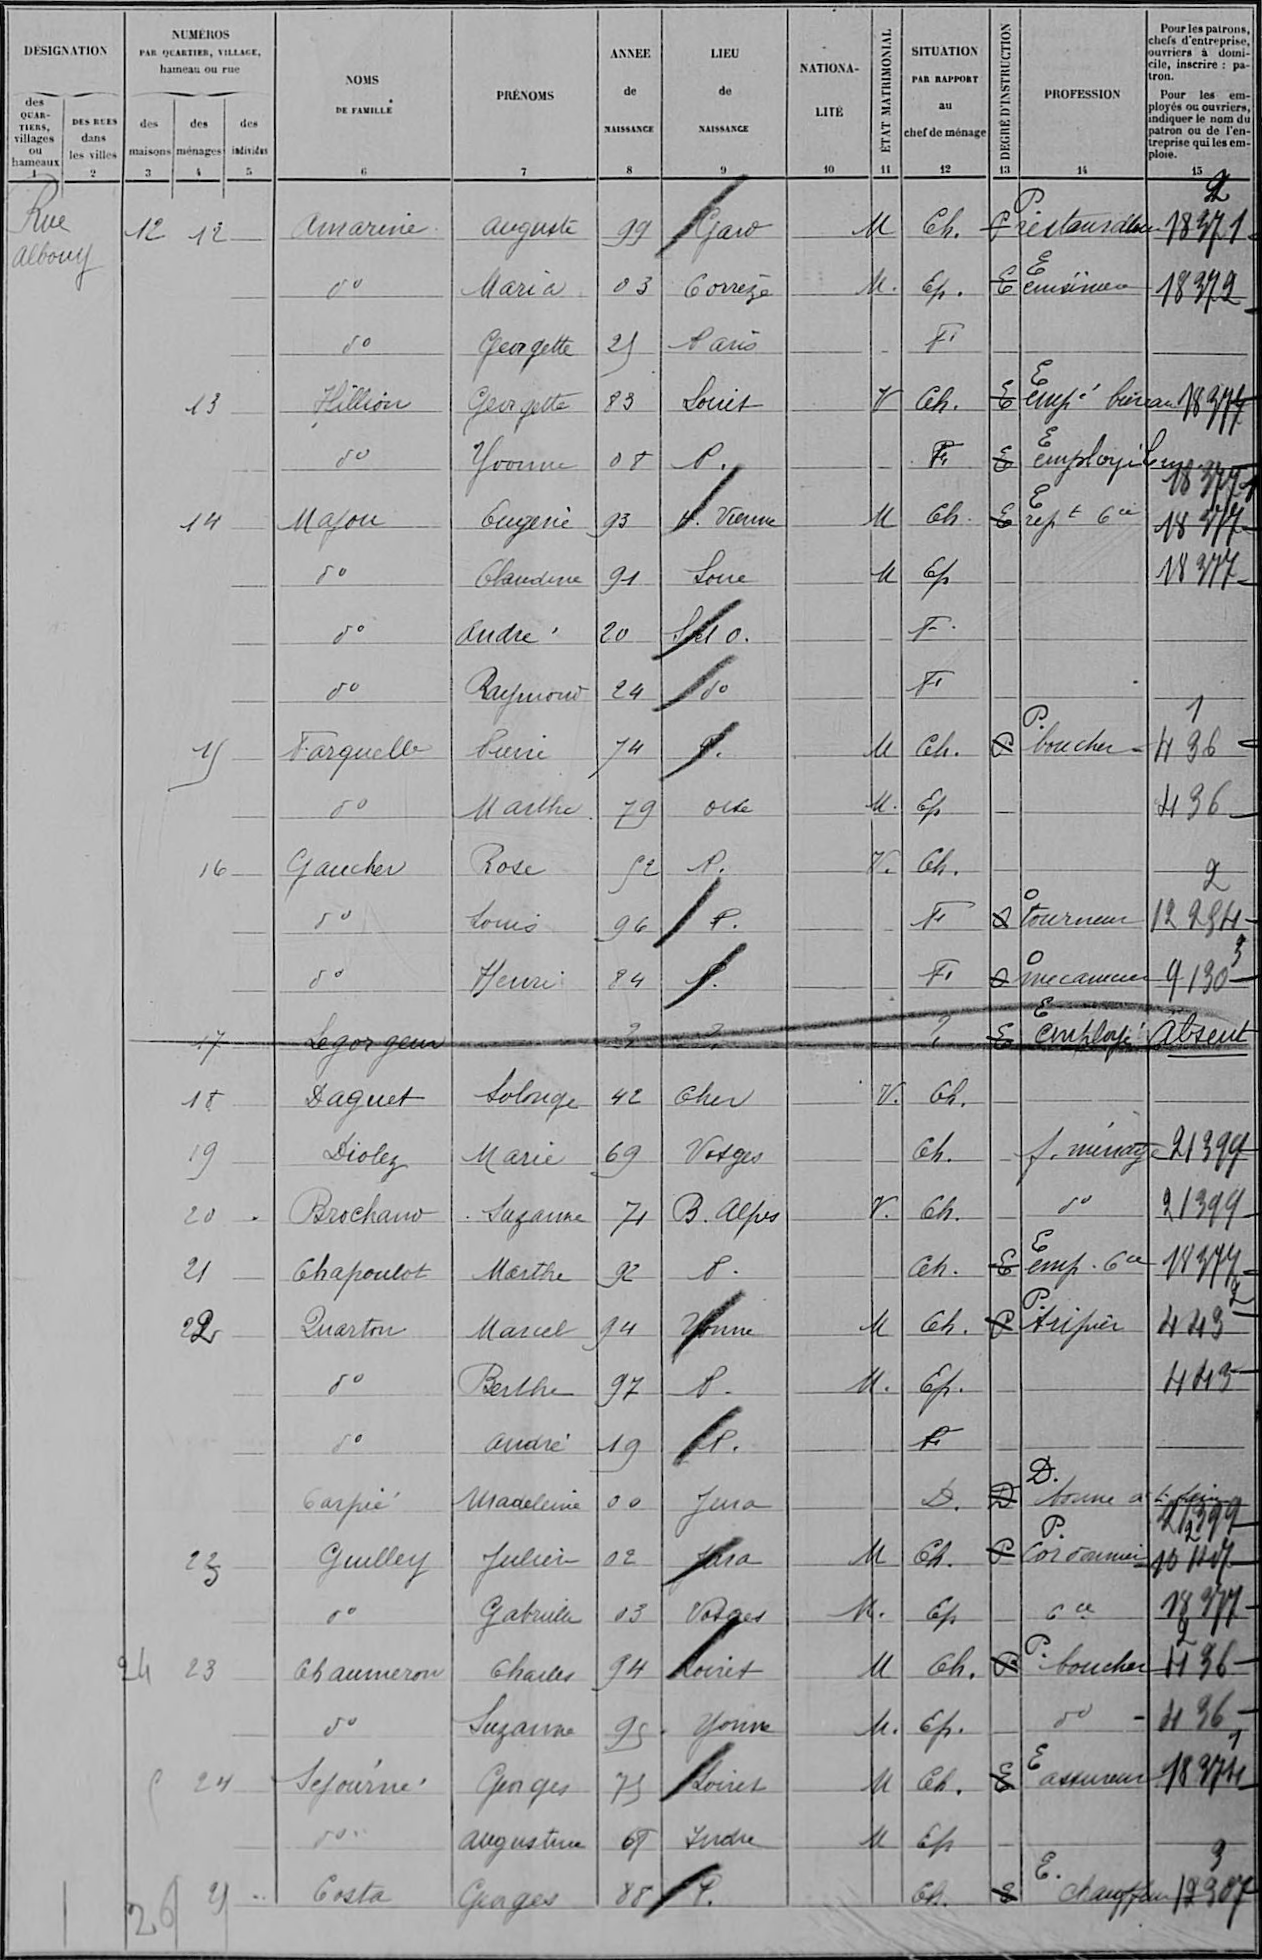Armarine/Auguste/99/Gard/¤/M/Ch /¤/P restaurateur/18371
d°/Maria/03/Corrèze/¤/M /Ep /¤/E cuisinière/18372
d°/Georgette/25/Paris/¤/¤/F/¤/¤/¤
Hillion/Georgette/83/Louis/¤/V/Ch /¤/E empé bureau/18377
d°/Yvonne/08/P /¤/¤/F/¤/E employée Cm/!18377
Majou/Eugène/93/H Vienne/¤/M/Ch /¤/E rept Cce/18377
d°/Claudine/91/Loire/¤/M/Ep/¤/¤/18377
d°/André/20/S et O /¤/¤/F/¤/¤/¤
d°/Raymond/24/d°/¤/¤/F/¤/¤/¤
Farquelle/Pierre/74/P /¤/M/Ch /¤/P boucher/436
d°/Marthe/79/Oise/¤/M /Ep/¤/¤/436
Gaucher/Rose/52/P /¤/V /Ch /¤/¤/¤
d°/Louis/96/P /¤/¤/F/¤/o tourneur/12254
d°/Henri/84/P /¤/¤/F /¤/o mecanicien/9130
Legorgeur/¤/¤/¤/¤/¤/¤/¤/E Employé/absent
Daguet/Solange/42/Cher/¤/V /Ch /¤/¤/¤
Diolez/Marie/69/Vosges/¤/¤/Ch /¤/f ménage/21399
Brochand/Suzanne/71/B Alpes/¤/V /Ch /¤/d°/21399
Chapoulot/Marthe/92/P /¤/¤/Ch /¤/E emp Cce/18377
Quarton/MArcel/94/Yonne/¤/M/Ch /¤/P tripier/443
d°/Berthe/97/P /¤/M /Ep /¤/¤/443
d°/André/10/P /¤/¤/f/¤/¤/¤
Tarpié/Madeleine/00/Jura/¤/¤/D /¤/D bonne à t faire/¤
Guilley/Julien/02/Jura/¤/M/Ch /¤/P cordonnier/10147
d°/Gabrielle/03/Vosges/¤/M /Ep/¤/cce/18377
Chaumeron/Charles/94/Loiret/¤/M/Ch /¤/P boucher/436
d°/Suzanne/95/Yonne/¤/M /Ep /¤/d°/436
Séjourné/Georges/75/Loiret/¤/M/Ch /¤/E assureur/18374
d°/augustine/68/Indre/¤/M/Ep/¤/¤/¤
Costa/Georges/88/P /¤/¤/Ch /¤/E chauffeur/12307
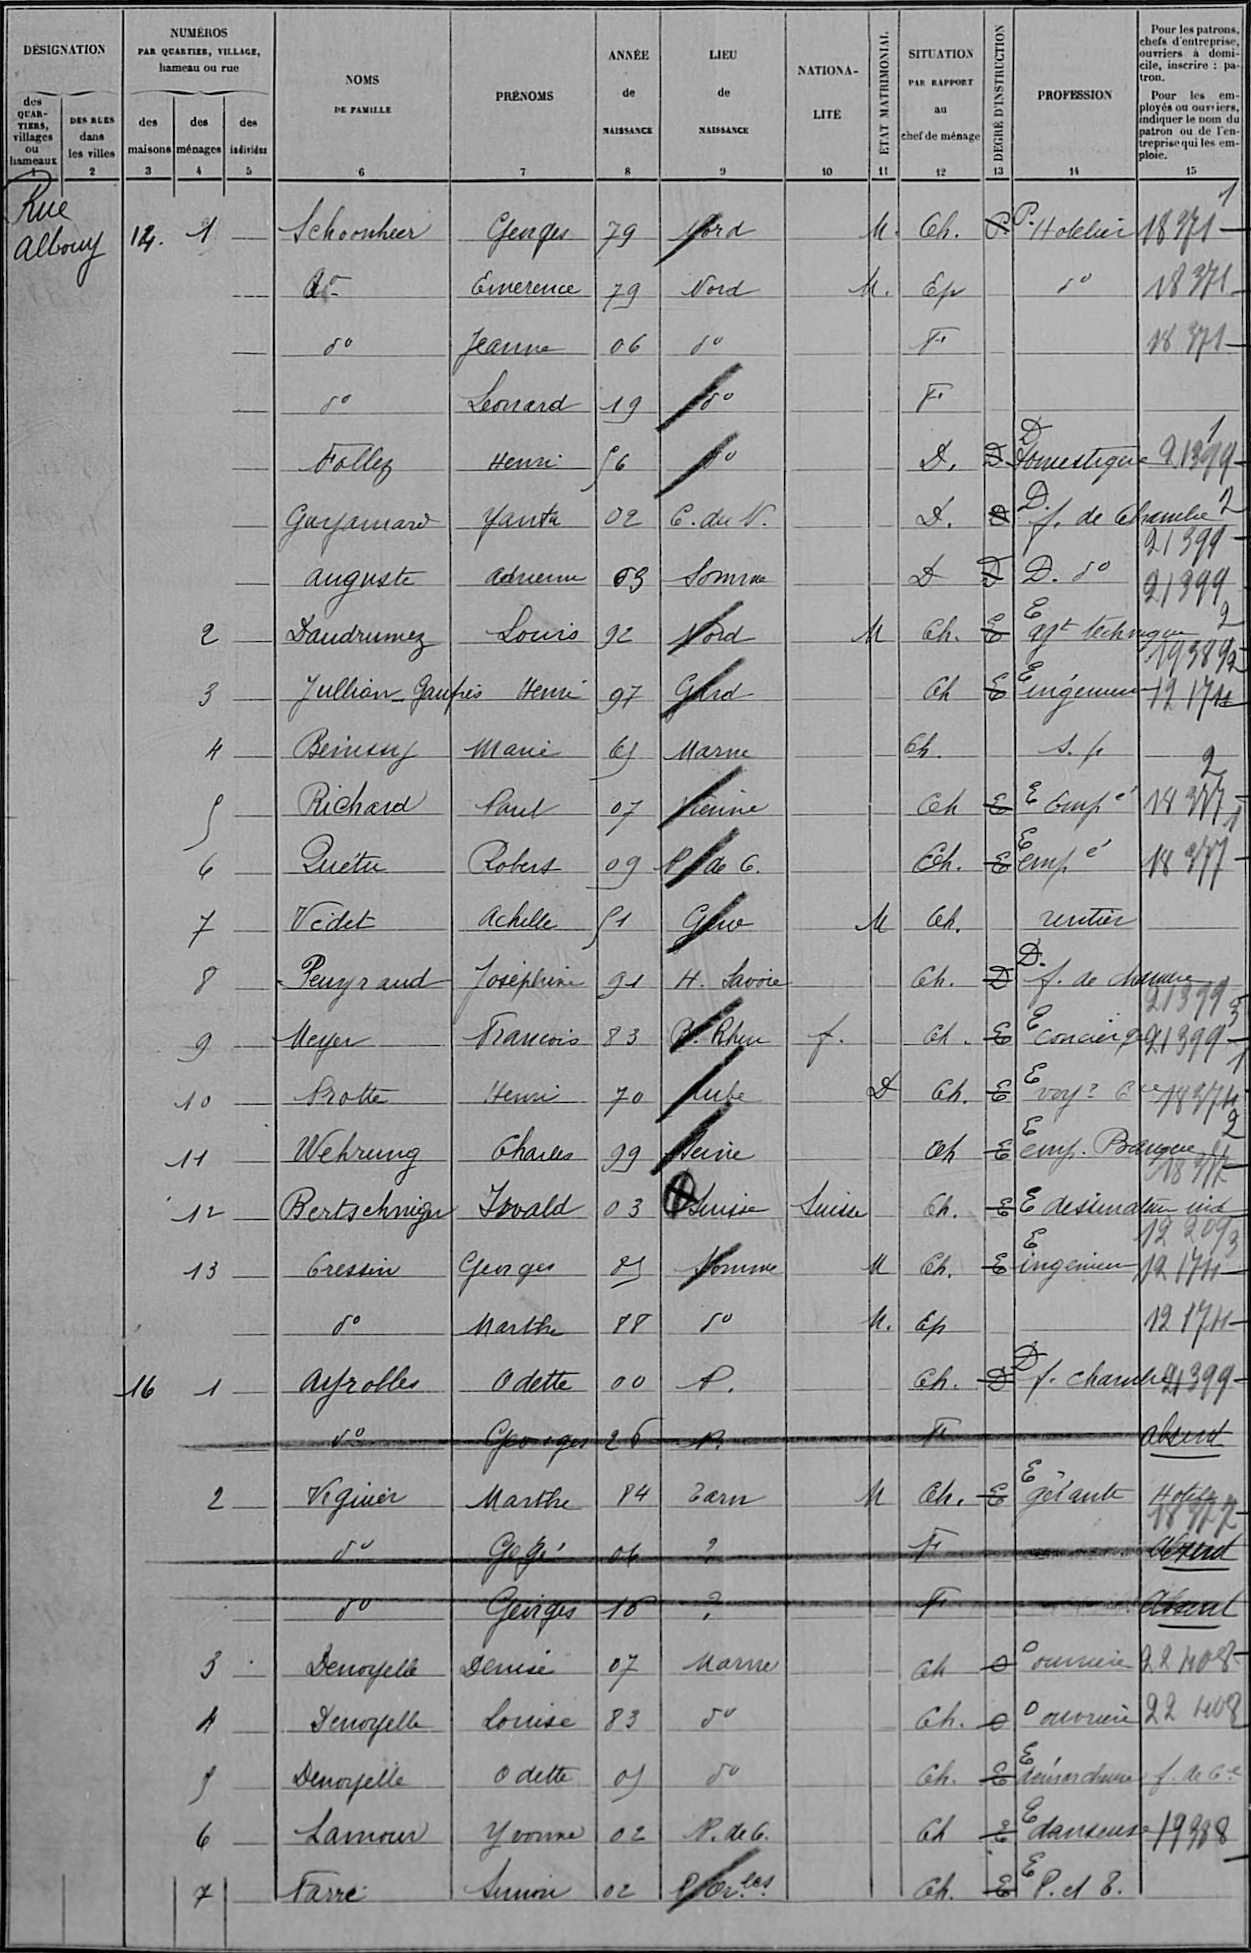Schoonheer/Georges/79/Nord/¤/M/Ch /¤/P Hotelier/18371
d°/Emerence/79/Nord/¤/M /Ep/¤/d°/18371
d°/Jeanne/06/d°/¤/¤/F/¤/¤/18371
d°/Leonard/19/d°/¤/¤/F/¤/¤/¤
Follez/Henri/56/d°/¤/¤/D /¤/Domestique/21399
Guyamard/Yanta/02/C du N /¤/¤/D /¤/D f de chambre/!21399
Auguste/Adrienne/03/Somme/¤/¤/D/¤/D d°/21399
Daudrunez/Louis/92/Nord/¤/M/Ch /¤/E agt technique/!19389
Jullian-Gaupès/Henri/97/Gard/¤/¤/Ch/¤/E ingénieur/12174
Beinsuy/Marie/65/Marne/¤/¤/Ch /¤/S p/¤
Richard/Paul/07/Vienne/¤/¤/Ch/¤/E Empé/18377
Quétu/Robert/09/P de C /¤/¤/Ch /¤/E empé/18377
Védet/Achille/51/Gers/¤/M/Ch /¤/rentier/¤
Peuyraud/Joséphine/91/H Savoie/¤/¤/Ch /¤/D f de chambre/!21399
Meyer/François/83/B Rhin/f /¤/Ch /¤/E concierge/21399
Protte/Henri/70/Aube/¤/D/Ch /¤/E voyr Cce/18374
Wehrung/Charles/99/Seine/¤/¤/Ch/¤/E emp Banque/18377
Bertschniger/Isvald/03/Suisse/Suisse/¤/Ch /¤/E dessinateur ind /!12209
Cressin/Georges/85/Somme/¤/M/Ch /¤/E ingénieur/12174
d°/Marthe/88/d°/¤/M /Ep/¤/¤/12174
Ayrolles/Odette/00/P /¤/¤/Ch /¤/D f chambre/21399
d°/Georges/26/P /¤/¤/F/¤/¤/absent
Viguier/Marthe/84/Tarn/¤/M/Ch /¤/E gérante Hotel/!18377
d°/Gégé/06/¤/¤/¤/F/¤/¤/absent
d°/Georges/16/¤/¤/¤/F/¤/¤/absent
Denoyelle/Denise/07/Marne/¤/¤/Ch/¤/o ouvrier/22408
Denoyelle/Louise/83/d°/¤/¤/Ch /¤/o ouvrier/22408
Denoyelle/Odette/05/d°/¤/¤/Ch /¤/E désusarchienne /f de Ce
Lamour/Yvonne/02/P de C /¤/¤/Ch/¤/E danseuse/19388
Farre/Simon/02/P Orles/¤/¤/Ch /¤/E P et T /¤